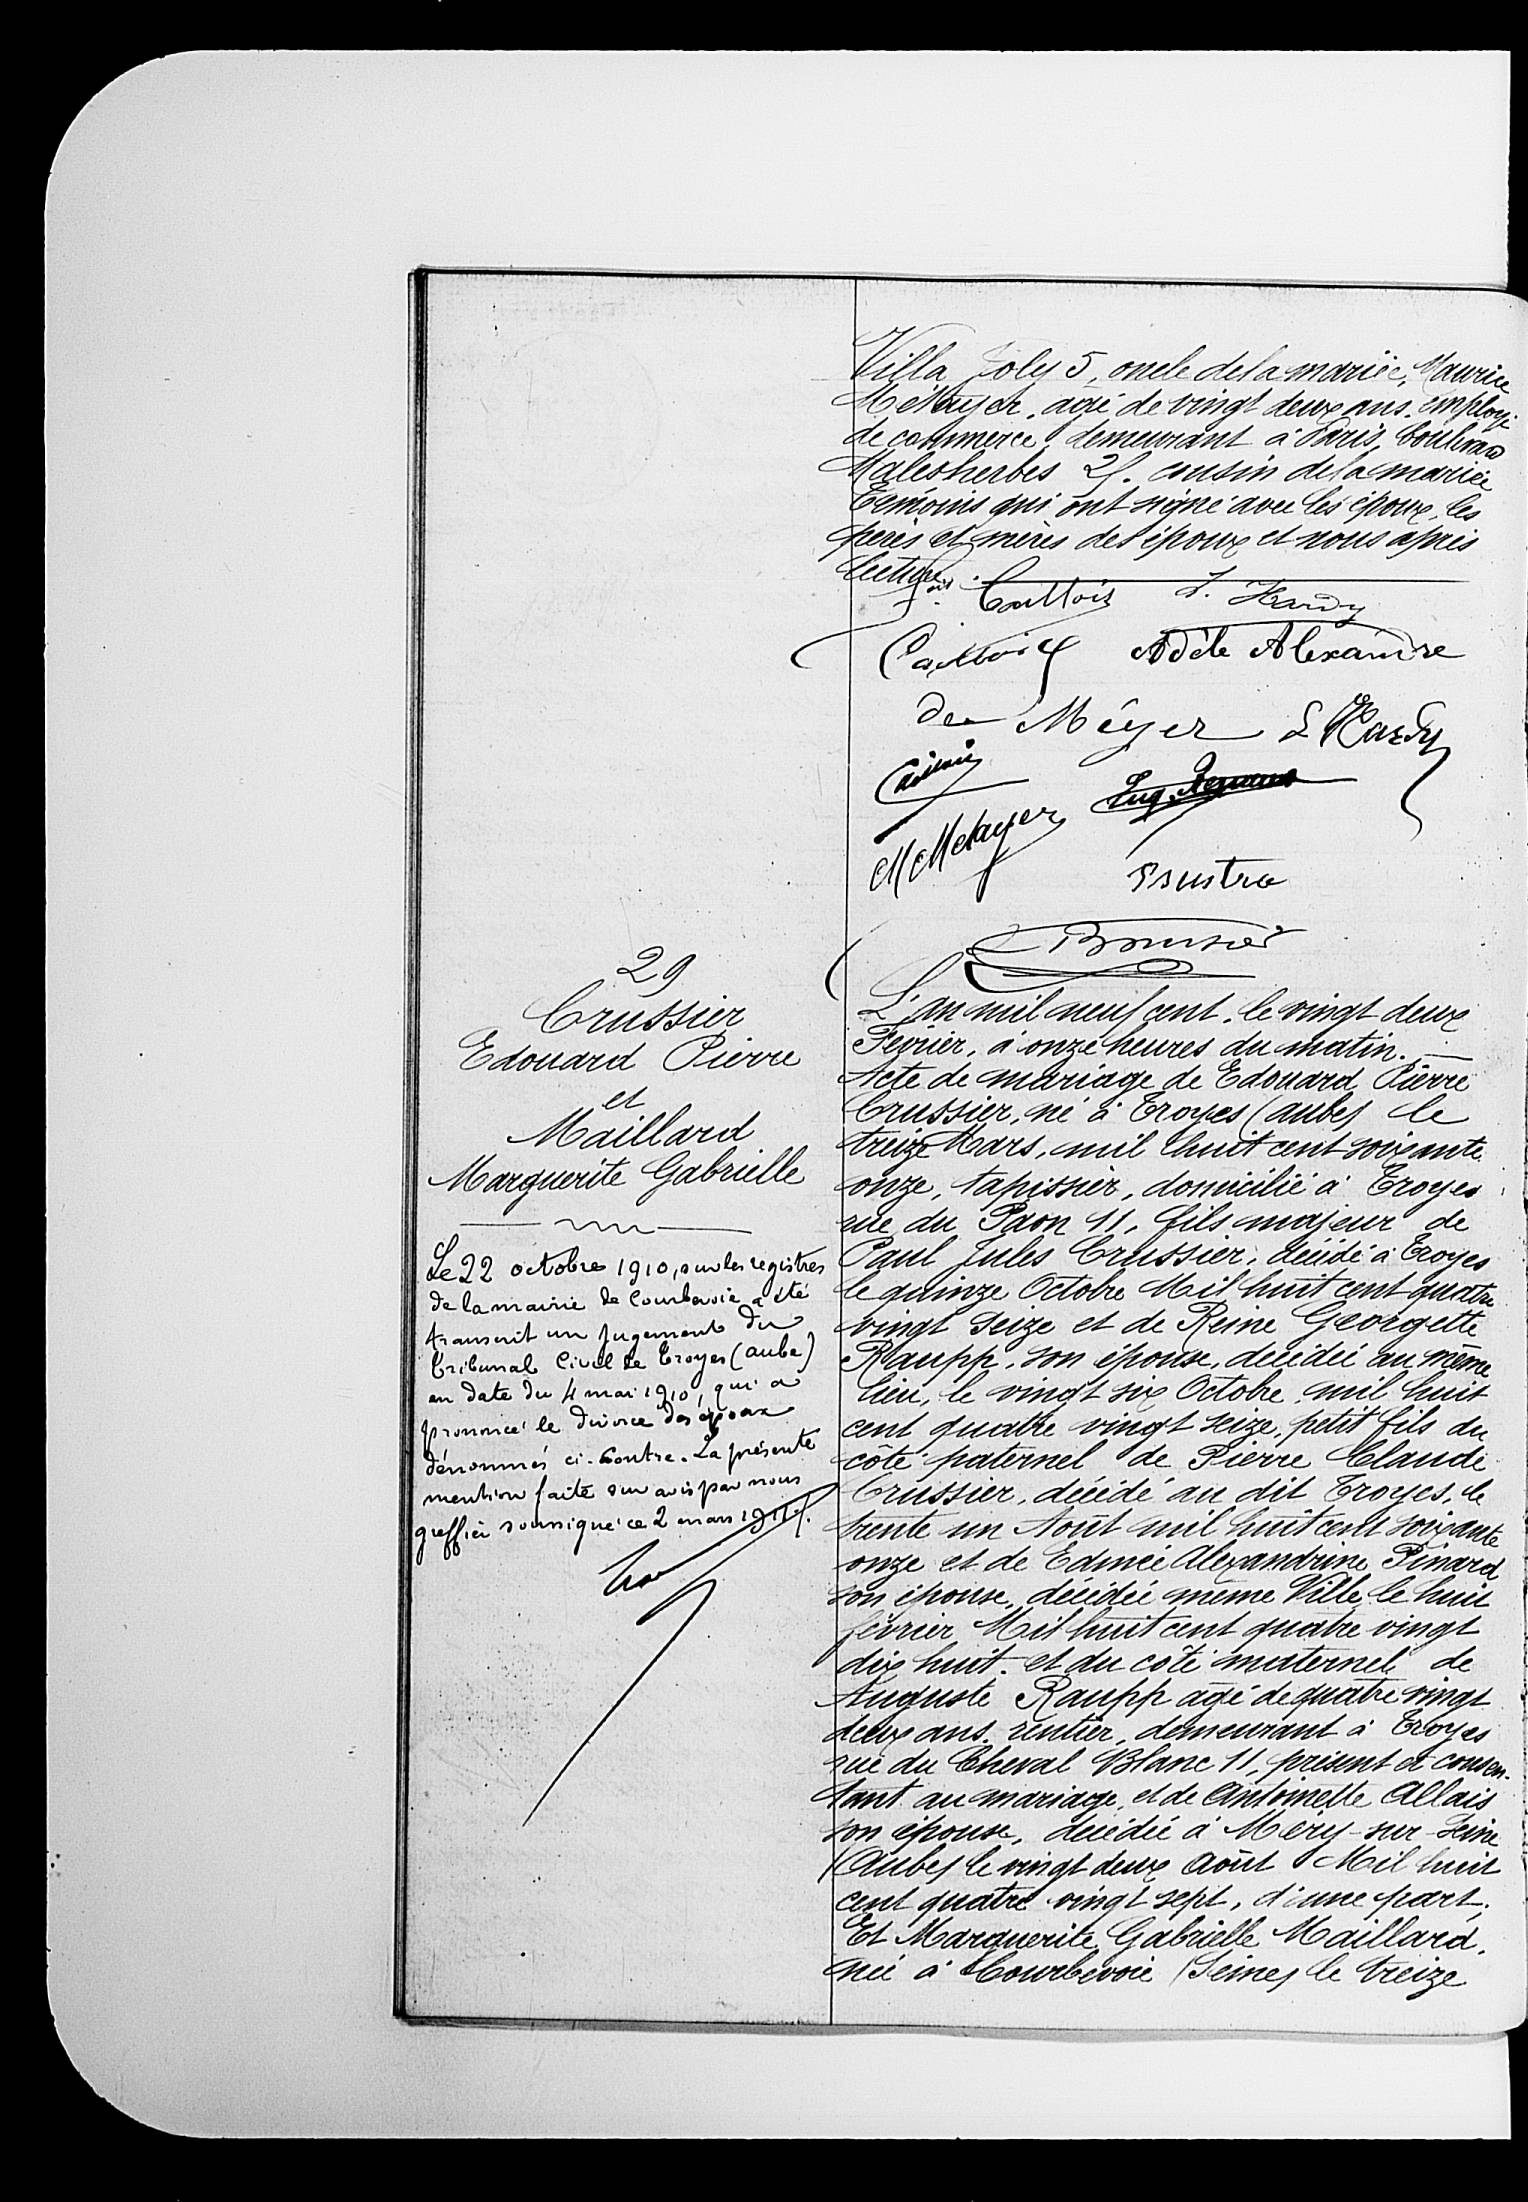villa Joly 5, oncle de la mariée, Maurice
Métayer, âgé de vingt deux ans, employé
de commerce demeurant à Paris, boulevard
Malesherbes 25, cousin de la mariée
témoins qui ont signé avec les époux, les
pères et mères des époux et nous après
lecture.
29
Crussier
Edouard Pierre
et
Maillard
Marguerite Gabrielle
L'an mil neuf cent, le vingt deux
Février, à onze heures du matin.-
Acte de mariage de Edouard Pierre
Crussier, né à Troyes (Aube) le
treize Mars, mil huit cent soixante
onze, tapissier, domicilié à Troyes
rue du Paon 11, fils majeur de
Paul Jules Crussier, décédé à Troyes
le quinze octobre mil huit cent quatre
vingt seize et de Reine Georgette
Raupp, son épouse, décédée au même
lieu, le vingt dix octobre mil huit
cent quatre vingt seize, petit fils du
côté paternel de Pierre Claude
Crussier, décédé au dit Troyes, le
trente un août mil huit cent soixante
onze et de Edmée Alexandrin Pinard
son épouse, décédée même Ville le huit
février mil huit cent quatre vingt
dix huit et du côté maternel de
Auguste Raupp âgé de quatre vingt
deux ans rentier, demeurant à Troyes
rue du Cheval Blanc 11, présent et consen-
tant au mariage et de Antoinette Allais
son épouse, décédée à Mery sur Seine
(Aube) le vingt deux août mil huit
cent quatre vingt sept, d'une part;
Et Marguerite Gabrielle Maillard,
née à Courbevoie (Seine) le treize
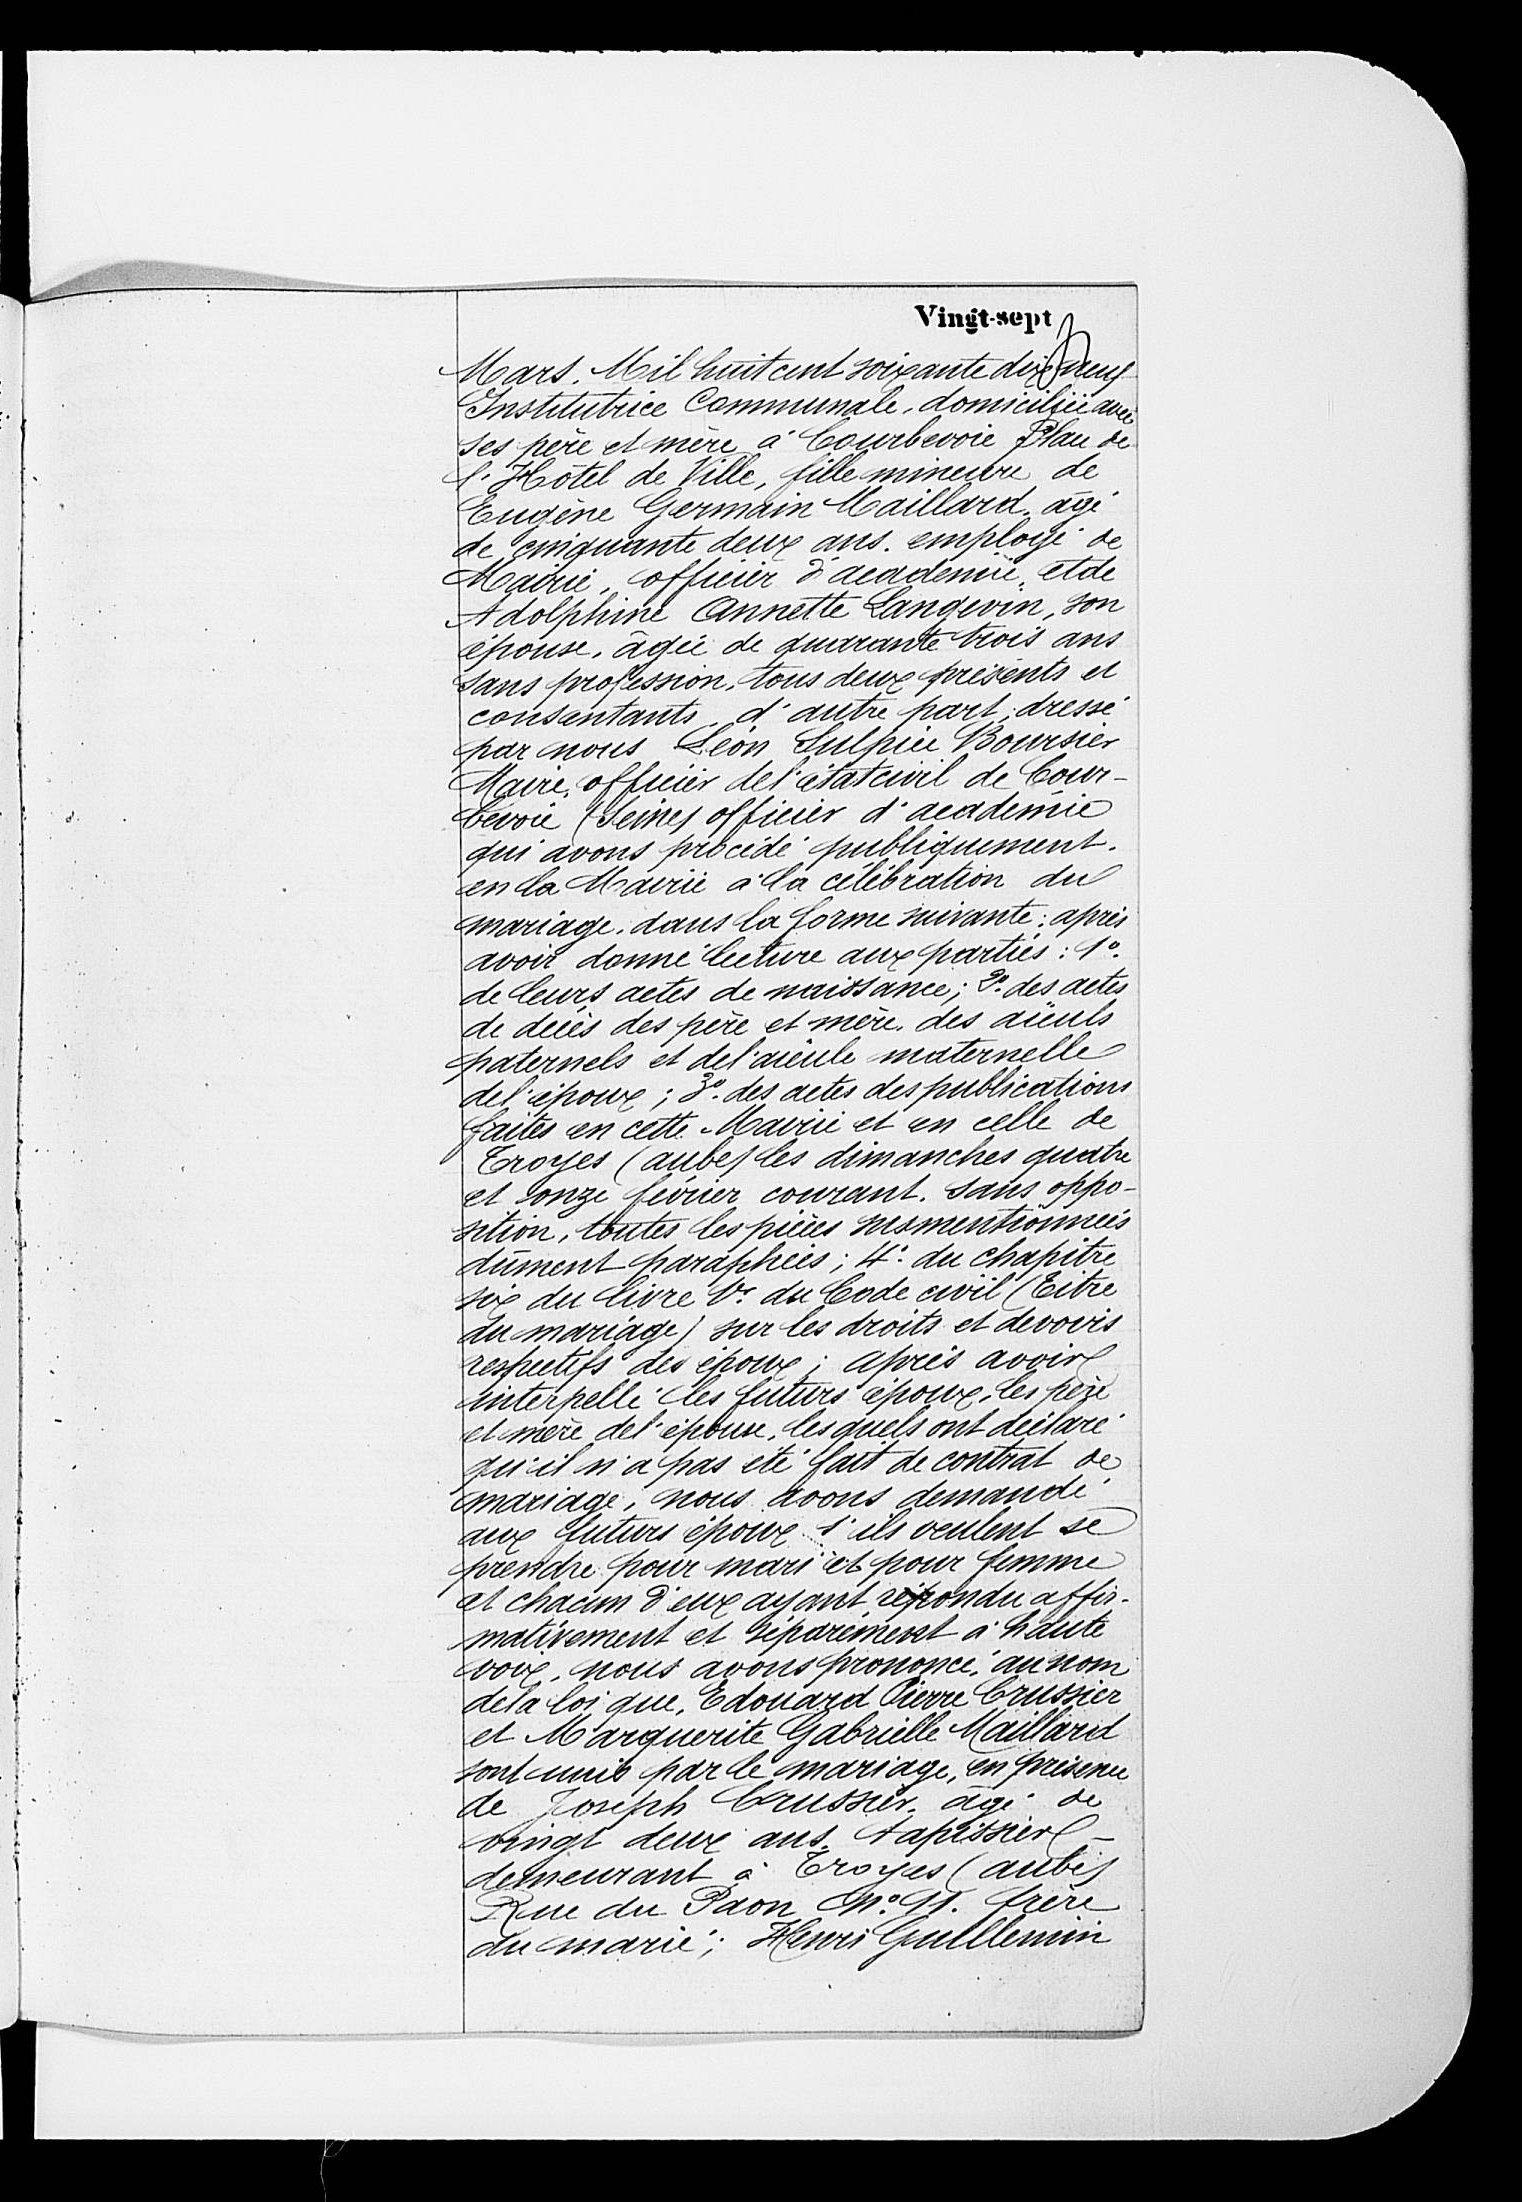Mar, Mil huit cent soixante dix neuf
Institutrice communale, domiciliée avec
ses père et mère à Courbevoie Place de
l'Hôtel de Ville, fille mineure de
Eugène Germain Maillard, âgé
de cinquante deux ans, employé de
Mairie, officier d'académie et de
Adolphine Annette Langevin, son
épouse, âgée de quarante trois ans
sans profession, tous deux présents et
consentants. d'autre part; dressé
par nous Léon Sulpice Boursier
Maire, officier de l'état civil de Cour-
bevoie (Seine) officier d'académie
qui avons procédé publiquement,
en la Mairie à la célébration du
mariage, dans la forme suivante: après
avoir donné lecture aux parties: 1°
de leurs actes de naissance; 2° des actes
de décès des père et mère des aïeuls
paternel et de l'aïeule maternelle
de l'épouse; 3° des actes des publications
faites en cette Mairie et un celle de
Troyes (Aube) les dimanches quatre
et onze février courant. sans opposition,
toutes les pièces susmentionnées
dûment paraphées; 4: du chapitre
six du livre 1er du Code civil (Titre
du mariage) sur les droits et devoirs
respectifs des époux; après avoir
interpellé les futurs époux, les père
et mère de l'épouse, lesquels ont déclaré
qu'il n'a pas été fait de contrat de
mariage, nous avons demandé
aux futurs époux s'ils veulent se
prendre pour mari et pour femme
et chacun d'eux ayant répondu affir-
mativement et séparément à haute
voix, nous avons prononcé au nom
de la loi que, Edouard Pierre Crussier
et Marguerite Gabrielle Maillard
sont unis par le mariage, en présence
de Joseph Crussier, âgé de
vingt deux ans, tapissier -
demeurant à Troyes (Aube)
Rue du Paon n°11 frère
du marié, Henri Guillemin